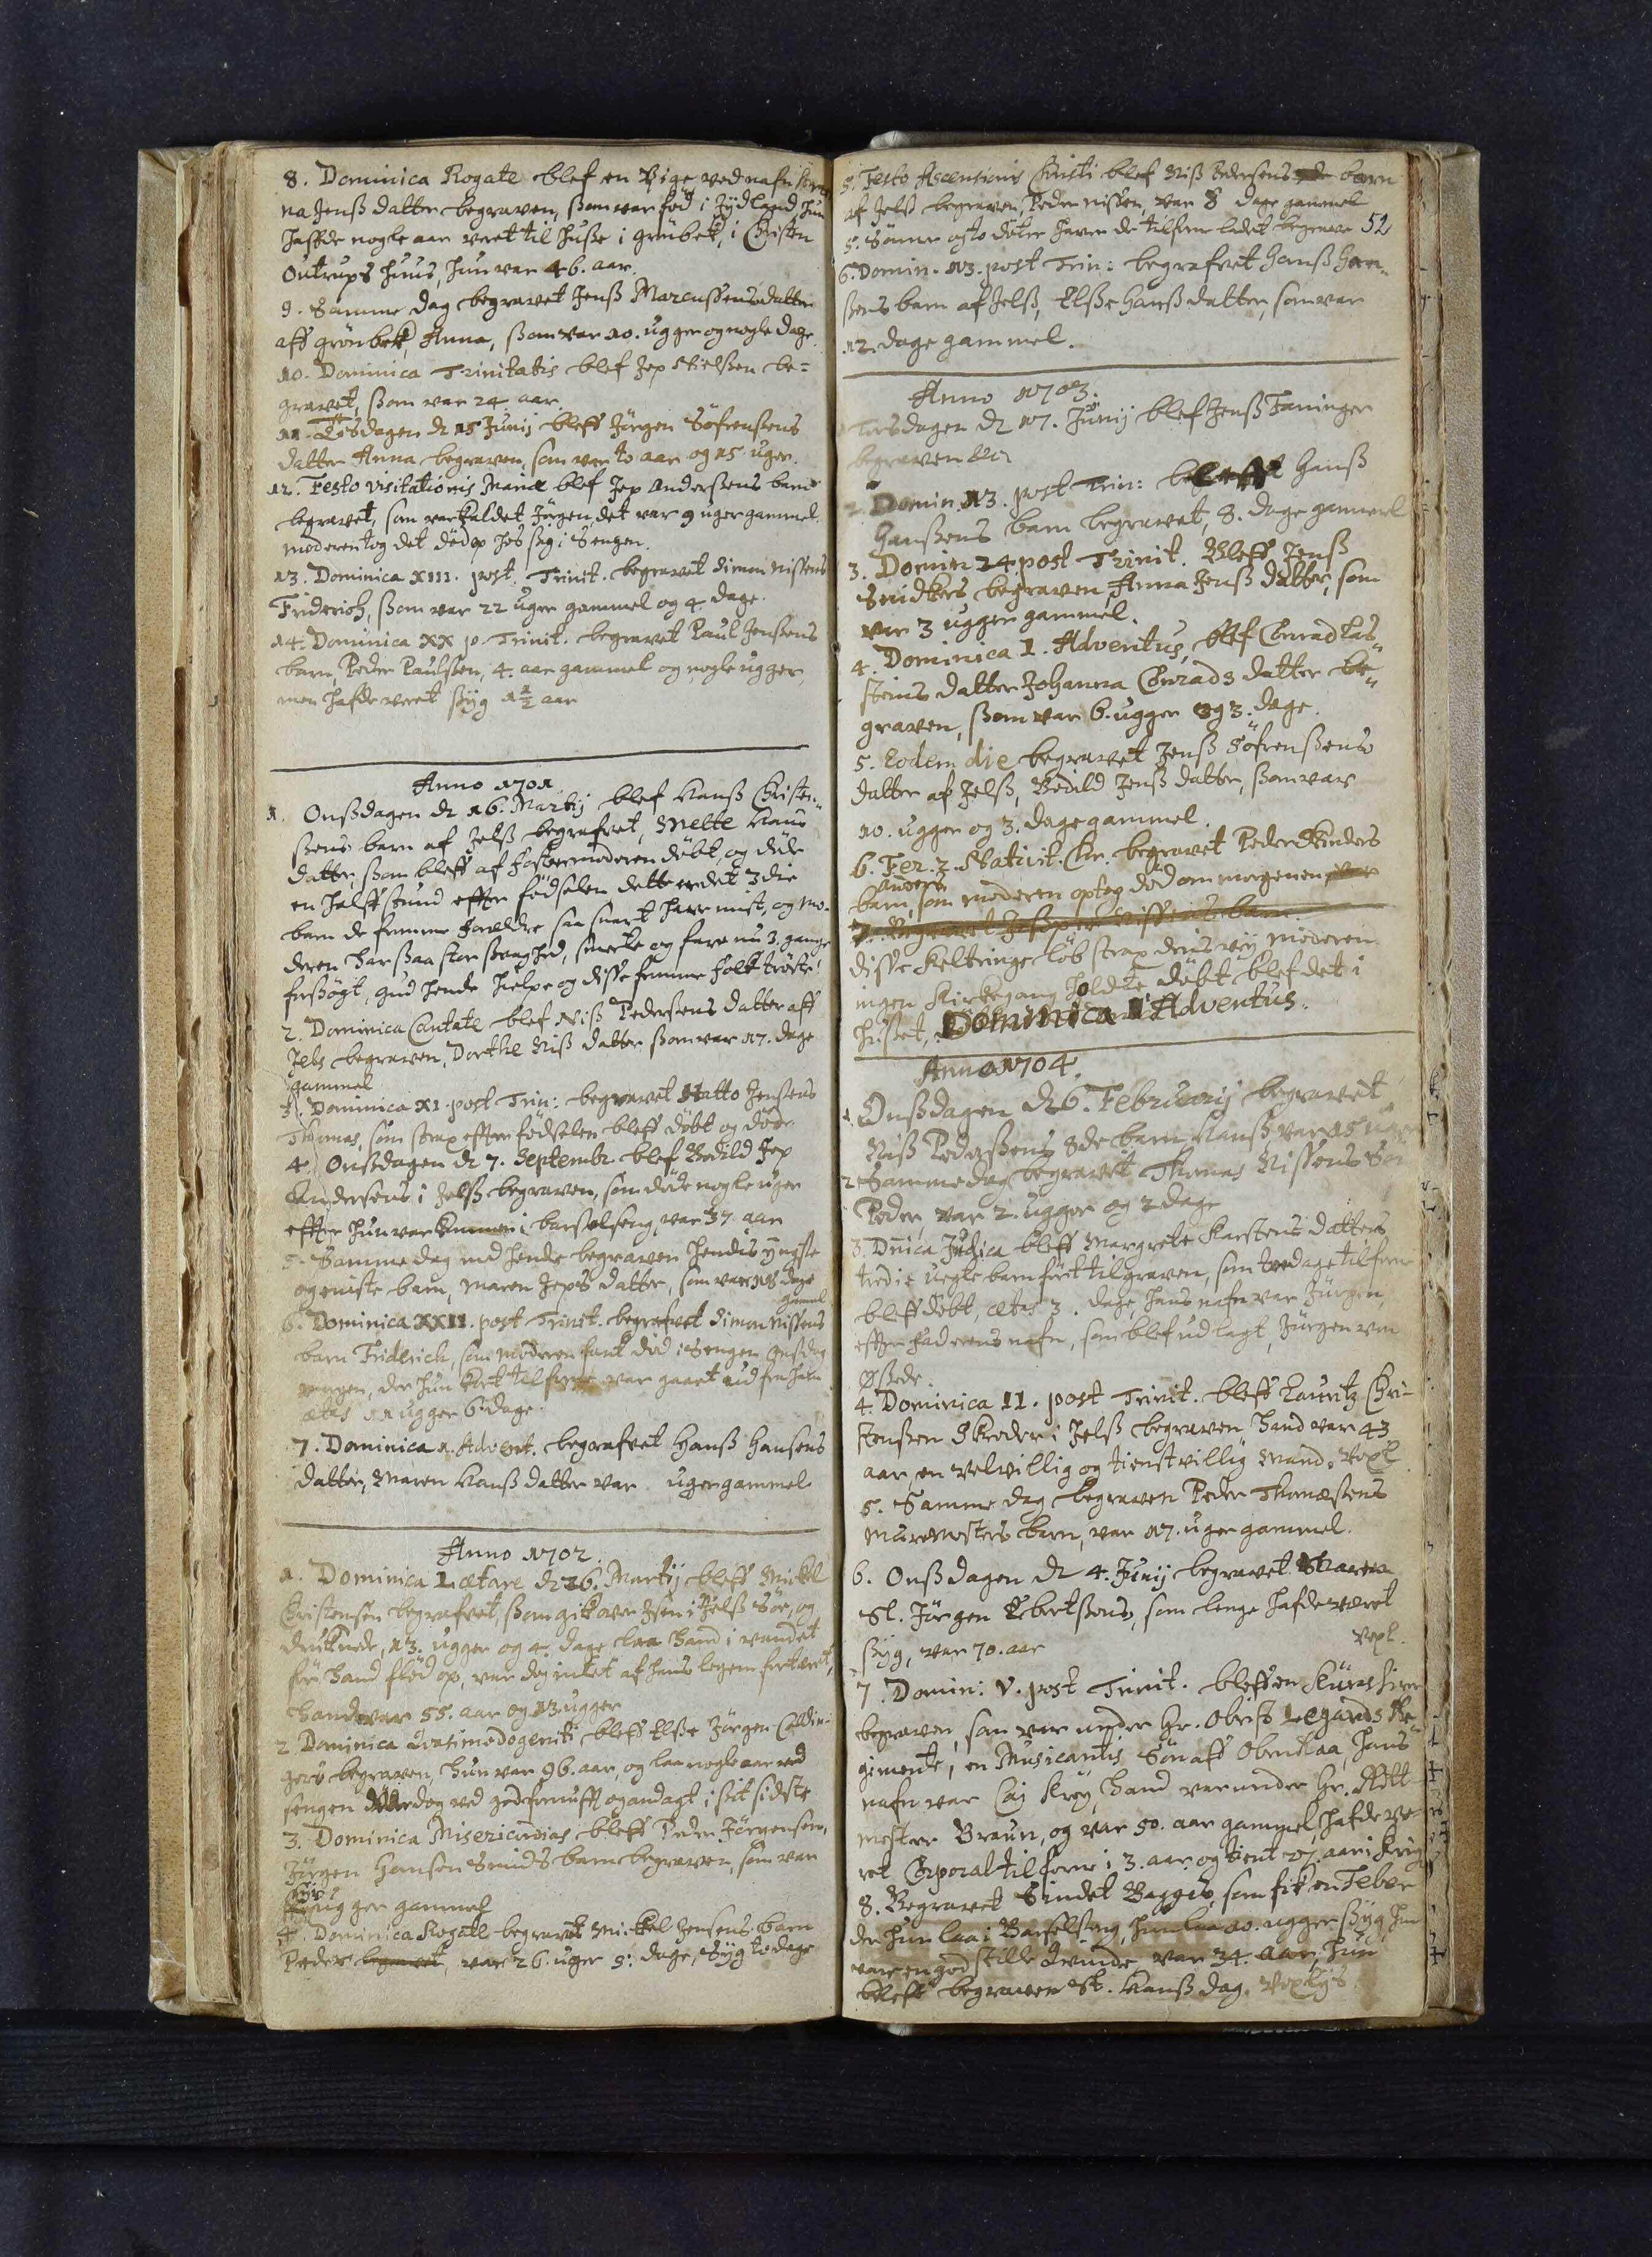8. Dominica Rogate blef en Pige ved nafn An¬
na Jens Datter begraven, ssom var fød i Jydland, hun
haffde nogle aar veret til husse i Grønbek, i Christen
Outrups huus, hun var 46. aar.
9. Samme Dag begravet Jenss Marcussens Datter
aff Grønbek, Anna, ssom var 10. ugger og nogle dage.
10. Dominica Trinitatis blef Jep Nielssen be
gravet, ssom var 24 aar.
11. Tisdagen d 15 Junij bleff Jørgen Søfrenssens
datter Anna Begraven, som var to aar og 15 uger.
12. Festo Visitationis Mariæ blef Jep Anderssens barn
begravet, som var kaldet Jørgen, det var 9 uger gammel
moderen tog det død op hos sig i Sengen.
13. Dominica XIII. Post Trinit. begravet Simon Nissens
Friderich, som var 22 uger gammel og 4 dage.
14. Dominica XX p. Trinit. begravet Paul Jenssens
barn, Peder Paulsen, 4. aar gammel og nogle ugger,
men hafde veret ssyg 1 ½ aar.
Anno 1701
1. Onsdagen d 16. Martij blef Hanss Christen¬
sens barn af Jelss begrafvet, Mette Hans
Datter, ssom bleff af fostermoderen døbt, og døde
en halff stund efter fødslen dette ere det 3die
barn de fromme Forældre saa snart have mist, og mo¬
deren har ssaa stor svaghed, smerte og fare nu 3. gange
forssøgt, Gud hende hielpe og disse fromme folk trøste!
2. Dominica Cantate blef Niss Pedersens datter aff
Jels begraven, Dorthe Niss Datter ssom var 17. Dage
gammel
3. Dominica XI. post Trin: begravet Hatto Jensens
Thomas, som strax efter fødslen bleff døbt og døde.
4. Onssdagen d 7. Septembr blef Bodild Jep
Andersens i Jelss begraven, som døde nogle uger
effter hun var kommem## i barselseng, var 37 aar
5. Samme dag ved hende begraven hendis yngste
og eniste barn, Maren Jeps datter, som var 18 dage
gammel
6. Dominica XXII. post Trinit begrafet Simon Nissens
barn Friderich, som moderen fant død i sengen Onsdag
morgen, der hun kort tilforn var gaaet ud fra ham
ætas 11 ugger 6 dage
7. Dominica 1. Advent. begrafvet Hanss Hansens
datter, Maren Hanss Datter var uger gammel
Anno 1702
1. Dominica Lætare d 26. Martij bleff Mickel
Christensen begrafet, ssom gik over Isen i Jelss Søe, og
druknede, 13. ugger og 4 Dage laa hand i vandet
før hand flød op, vor dog intet af hans legem fortæret,
hand var 55. aar og 13. ugger
2. Dominica Qvasimodogeniti bleff Elsse Jørgen Coldin¬
gers begraven, hun var 96. aar, og laa nogle aar ved
sengen var## dog ved gtod fornufft og andagt i ssit sidste
3. Dominica Misericordias bleff Peder Jørgensen,
Jørgen Hansen Smids barn begraven, som var
syv
ugger gammel
4. Dominica Rogate begravet Mickel Jensens barn
Peder begravet, var 26. uger 5: dage, Syg to dage
52
5. Festo Ascensions Christi blef Niss Pedersens ##e barn
af Jelss begraven, Peder Nissen, var 8 Dage gammel
5. Sønner og to døtre haver de tilforn ladet begrave
6. Domin. 13. post Trin: begrafvet Hanss Han¬
sens barn af Jelss, Elsse Hanss datter, som var
12 dage gammel.
Anno 1703.
1. Torsdagen d 17. Junij blef Jenss Faninger
begraven
2. Domin. 13. post Trin. bleff Hanss
Hanssens barn begravet, 8. dage gammel
3. Domin. 24. post Trinit. Bleff Jenss
Snidkers, begraven, Anna Jenss Datter, som
var 3 ugger gammel.
4. Dominica 1. Adventus blef Conrad Las¬
steins datter Johanna Conrads datter be¬
graven, ssom var 6. ugger og 3. Dage
5. Eodem die begravet Jenss Søfrenssens
datter af Jelss, Bodild Jenss datter, ssom var
10. ugger og 3. dage gammel
6. Fer. 2. Nativit. Chr. begravet Peder Skreders
Anders
barn, som moderen optog død om morgenen
7. Begravet Jessper Nissens barn.
disse keltringe løb strax deris vey moderen
ingen kirkegang holdte døbt blef det i
huset, Dominica 1 Adventus.
Anno 1704.
1. Onssdagen d 6. Februarij begravet
Niss Pederssens 8de barn Hanss var 15
2. Samme dag begravet Thomas Nissens Søn
Peder, var 2. ugger og 2 dage
3. Dnica Judica bleff Margrete Karstens Datters
tredje uegte barn ført til graven, som to dage tilforne
blef døbt, ætes## 3. dage, hans nafn var Jurgen,
effter faderens nafn, som blef udlagt, Jurgen von
Øssede
4. Dominica II. post Trinit. bleff Lauritz Chri¬
stenssen Skreder i Jelss begraven hand var 43
aar, en velvillig og tienstvillig mand. Woxl.
5. Samme dag begraven Peder Thomæssens
Muremesters barn, var 17 uger gammel.
6. Onssdagen d 4. Junij begravet Maren
Sl. Jørgen Ebertssens, som lenge hafde været
ssyg, var 70. aar. Voxl.
7. Domin. V. post Trinit. bleff en kurassirer
begraven, som var under Hr. Obrist Legards Re¬
gimente, en Musicantis Søn aff Obenraa, hans
nafn var Caj Krøy, hand var uder Hr. Ritt¬
mester Braun, og var 50. aar gammel, hafde ve¬
ret Corporal tilforne i 3. aar. og tient 27. aar i Krig.
8. Begravet Sindet Bagges, som fik en Feber
da hun laa i Barselseng, hun laa 10. ugger ssyg hun
var en god stille Qvinde, var 34. aar, hun
bleff begraven St. Hanss dag. Woxlys.
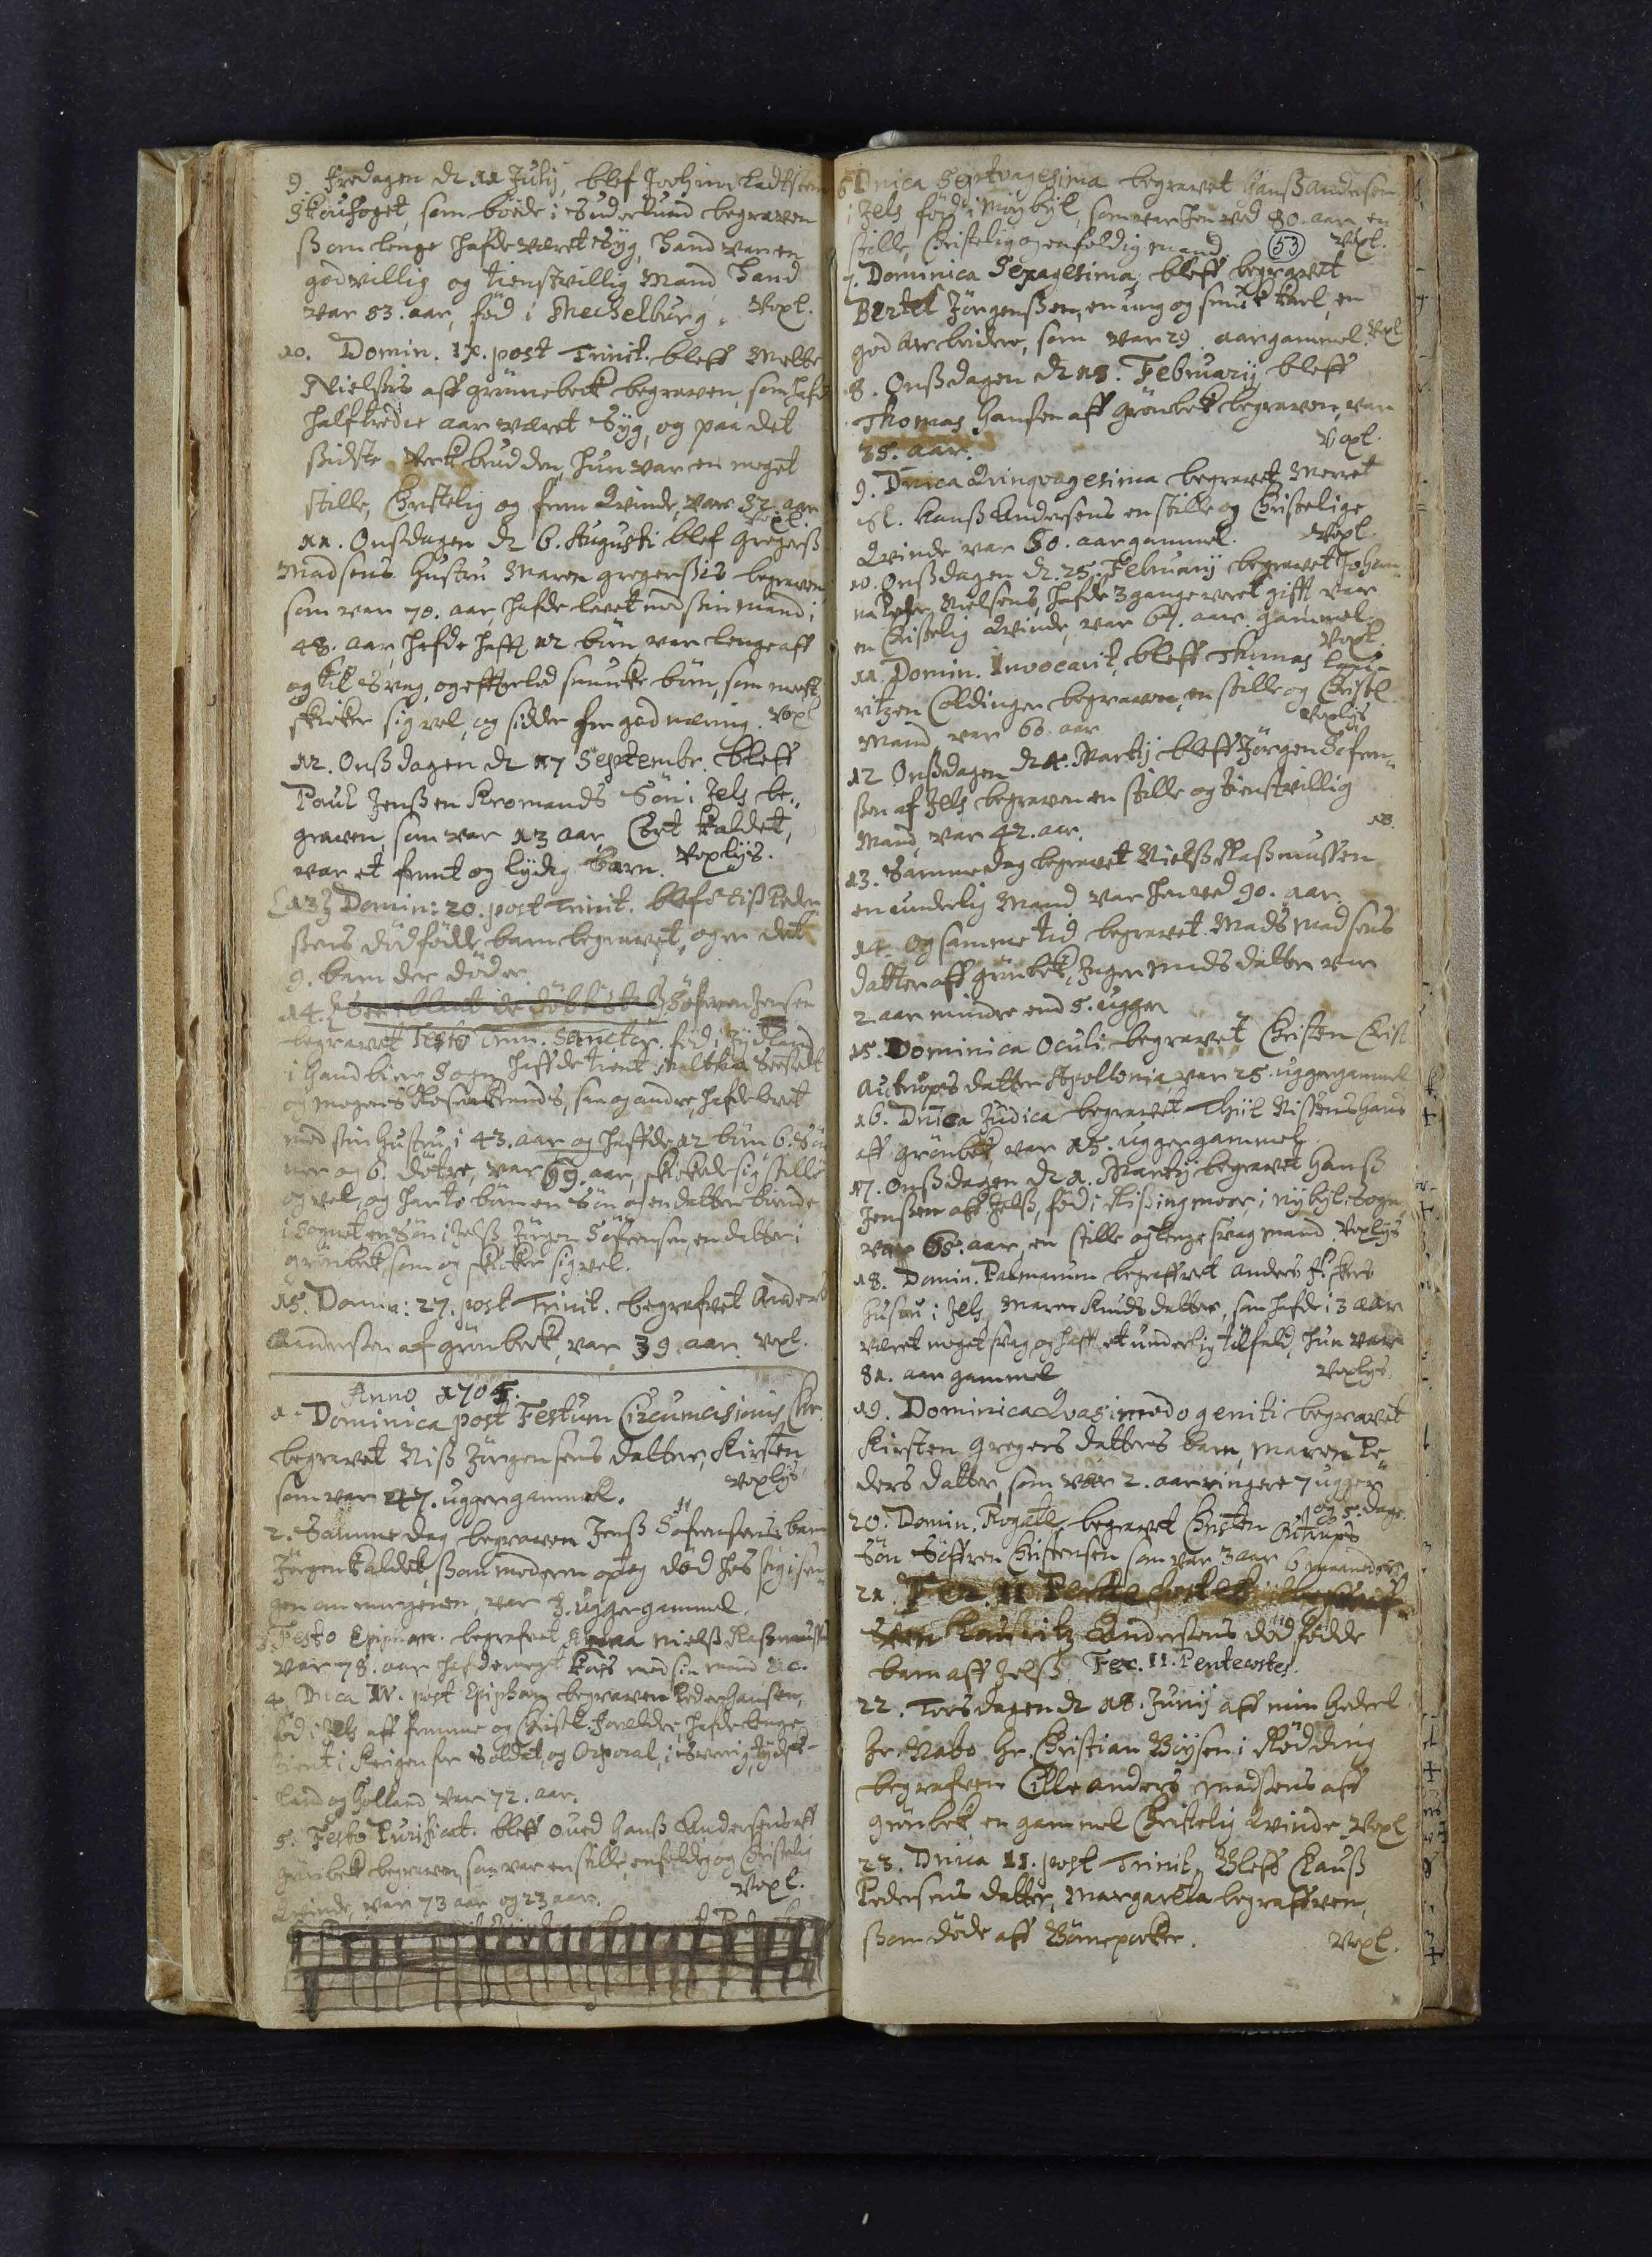9. Fredagen d 11 Julij blef Jochim Lasstein
Skoufoget, som boede i Suderlund begraven 
ssom lenge hafde veret Syg, hand var en
god villig og tienstvillig Mand hand
var 83. aar, fød i Mechelburg. Woxl.
10. Domin. IX. post Trinit. bleff Mette
Nielssis aff Grønnebeck begraven, som hafde
halftredie aar været Syg, og paa det 
sidste verkbrudden, hun var en meget 
stille, Christelig og from Qvinde, var 52. aar
Woxl.
11. Onssdagen d 6. Augusti blef Gregerss
Madsens Hustru Maren Gregerssis begraven
som var 70. aar hafde levet med ssin mand i
48. aar hafde hafft 12 børn, var lenge aff
og til Svag##, og efterlod smucke børn, som
skicke sig vel, og sidder for god næring. Woxl.
12. Onssdagen d 17. Septembr bleff
Poul Jenssen Kromands Søn i Jels be¬
graven, som var 13 aar, Cort kaldet,
var et fromt og lydig barn. Woxlys.
{13} Domin: 20. post Trinit. blef Niss Peder¬
ssens dødfødde barn begravet, og## det
9. barn der døde
14. {S## ## de døbte ##}. Søfren Jensen
begravet Festo Trin. Sanctor. fød i Jydland
i Handbierg Sogn hafde tient Matthis 
og Mogens Ros##ken##eds, saa og andre, hafde levet
med sin Hustru i 43. aar og haffde 12 børn 6. søn¬
ner og 6. døtre, var 69. aar, skickede sig stille
og vel, og har to børn en søn og en datter boende
i Sognet en Søn i Jelss Jørgen Søfrensen, en datter i
Grønbeck, som og skicker sig vel.
15. Domin: 27. post Trinit. begrafvet Anders
Andersen aff Grønbeck, var 39. aar. Woxl.
Anno 1705
1. Dominica post Festum Circumcisionis Chr.
begravet Niss Jørgensens datter, Kirsten
som var 47. ugger gammel.
Woxlys
2. Samme Dag begraven Jenss Søfrensens barn
Jørgen kaldet, ssom moderen optog død hos sig i sen¬
gen om morgenen, var 3. ugger gammel.
3. Festo Epiphan. Begrafvet Anna Nielss
var 78. Aar hafde meget k##s med sin mand ##c.
4. Dnca IV. post Epiphan begraven Peder Hansen,
fød i Jels aff fromme og Christl. Forældre; hafde lenge
tient i Krigen for Soldat, og Ocporal, i Sverrig, Tydsk¬
land og Holland## var 72. aar.
5. Festo Purificat. bleff Oued Hanss Andersens aff
Grønbek begraven, som var en stille, enfoldig og Christelig
Qvinde, var 73 aar og 23 aar.
Woxl.
53
6. Dnica Septuagesima begravet Hanss Andersen
i Jels, fød i Moybyl, som var hen ved 80. aar, en
stille, Christelig og enfoldig mand.
Woxl.
7. Dominica Sexagesima bleff begravet
Bertel Jørgenssen , en ung og smuck Karl, en
god arbeider, som var 29. aar gammel. Woxl.
8. Onssdagen d 18. Februarij bleff
Thomas Hansen aff Grønbek begraven, var
35. aar.
Woxl.
9. Dnica Qvinqvagesima begravet Merret
Sl. Hanss Andersens en stille og Christelige
Qvinde var 60. aar gammel. Woxl
10. Onssdagen d. 25. Februarij begravet Johan¬
ne Peder Nielsens, hafde 3 gange veret gifft, var
en Christelig Qvinde, var 67. Aar. Gammel.
Woxl.
11. Domin. Invocavit bleff Thomas Lauri¬
tzen Coldinger begraven, en stille og Christl
Mand var 60. aar
Woxlys.
12. Onssdagen d 4. Martij bleff Jørgen Søfren¬
ssen af Jels begraven en stille og tienstvillig
mand, var 42. aar
NB.
13. Samme dag begravet Nielss Rassmussen
en underlig Mand var henved 90. aar.
14. Og samme tid begravet Mads Madsens
datter aff Grønbek, Inger Madsdatter var
2 aar mindre end 5. ugger
15. Dominica Oculi begravet Christen Christ
Autrupes datter Apollonia var 25. ugger gammel
16. Dnica Judica begravet Thiil Nissens Hans
aff Grønbek, var 15. ugger gammel
17. Onssdagen d 1. Martij begravet Hanss
Jenssen aff Jelss, fød i Rissingmoor i Nybyl Sogn.
var 65. aar, en stille og lenge svag mand. Woxlys.
18. Domin. Palmarum begraffvet Anders Fiskers
Hustru i Jels, Maren Knuds datter, som hafde i 3 aar
veret meget svag, og haft et underlig tilfald, hun var
81. aar gammel.
Woxlys.
19. Dominica Qvasimodogeniti begravet
Kirsten Gregers datters barn, Maren Pe¬
ders datter, som var 2. aar ringere 7 ugger
pg 5. dage
20. Domin. Rogate begravet Christen Outrups
søn Søffren Christensen som var 3 aar 6 maaneder.
21. Fer. II Pentecost begrafet
Lauritz Andersens dødfødde
barn aff Jelss. Fer. II. Pentecostes.
22. Torsdagen d 18. Junij aff min Hederl
Hr. Nabo Hr. Christian Boysen i Rødding
begrafen Cille Anders Madsens aff
Grønbek, en gammel Christelig Qvinde. Woxl
23. Dnica II. post Trinit. Bleff Clauss
Pedersens datter, Margareta begraffven,
ssom døde aff Børnepocker.
Woxl.